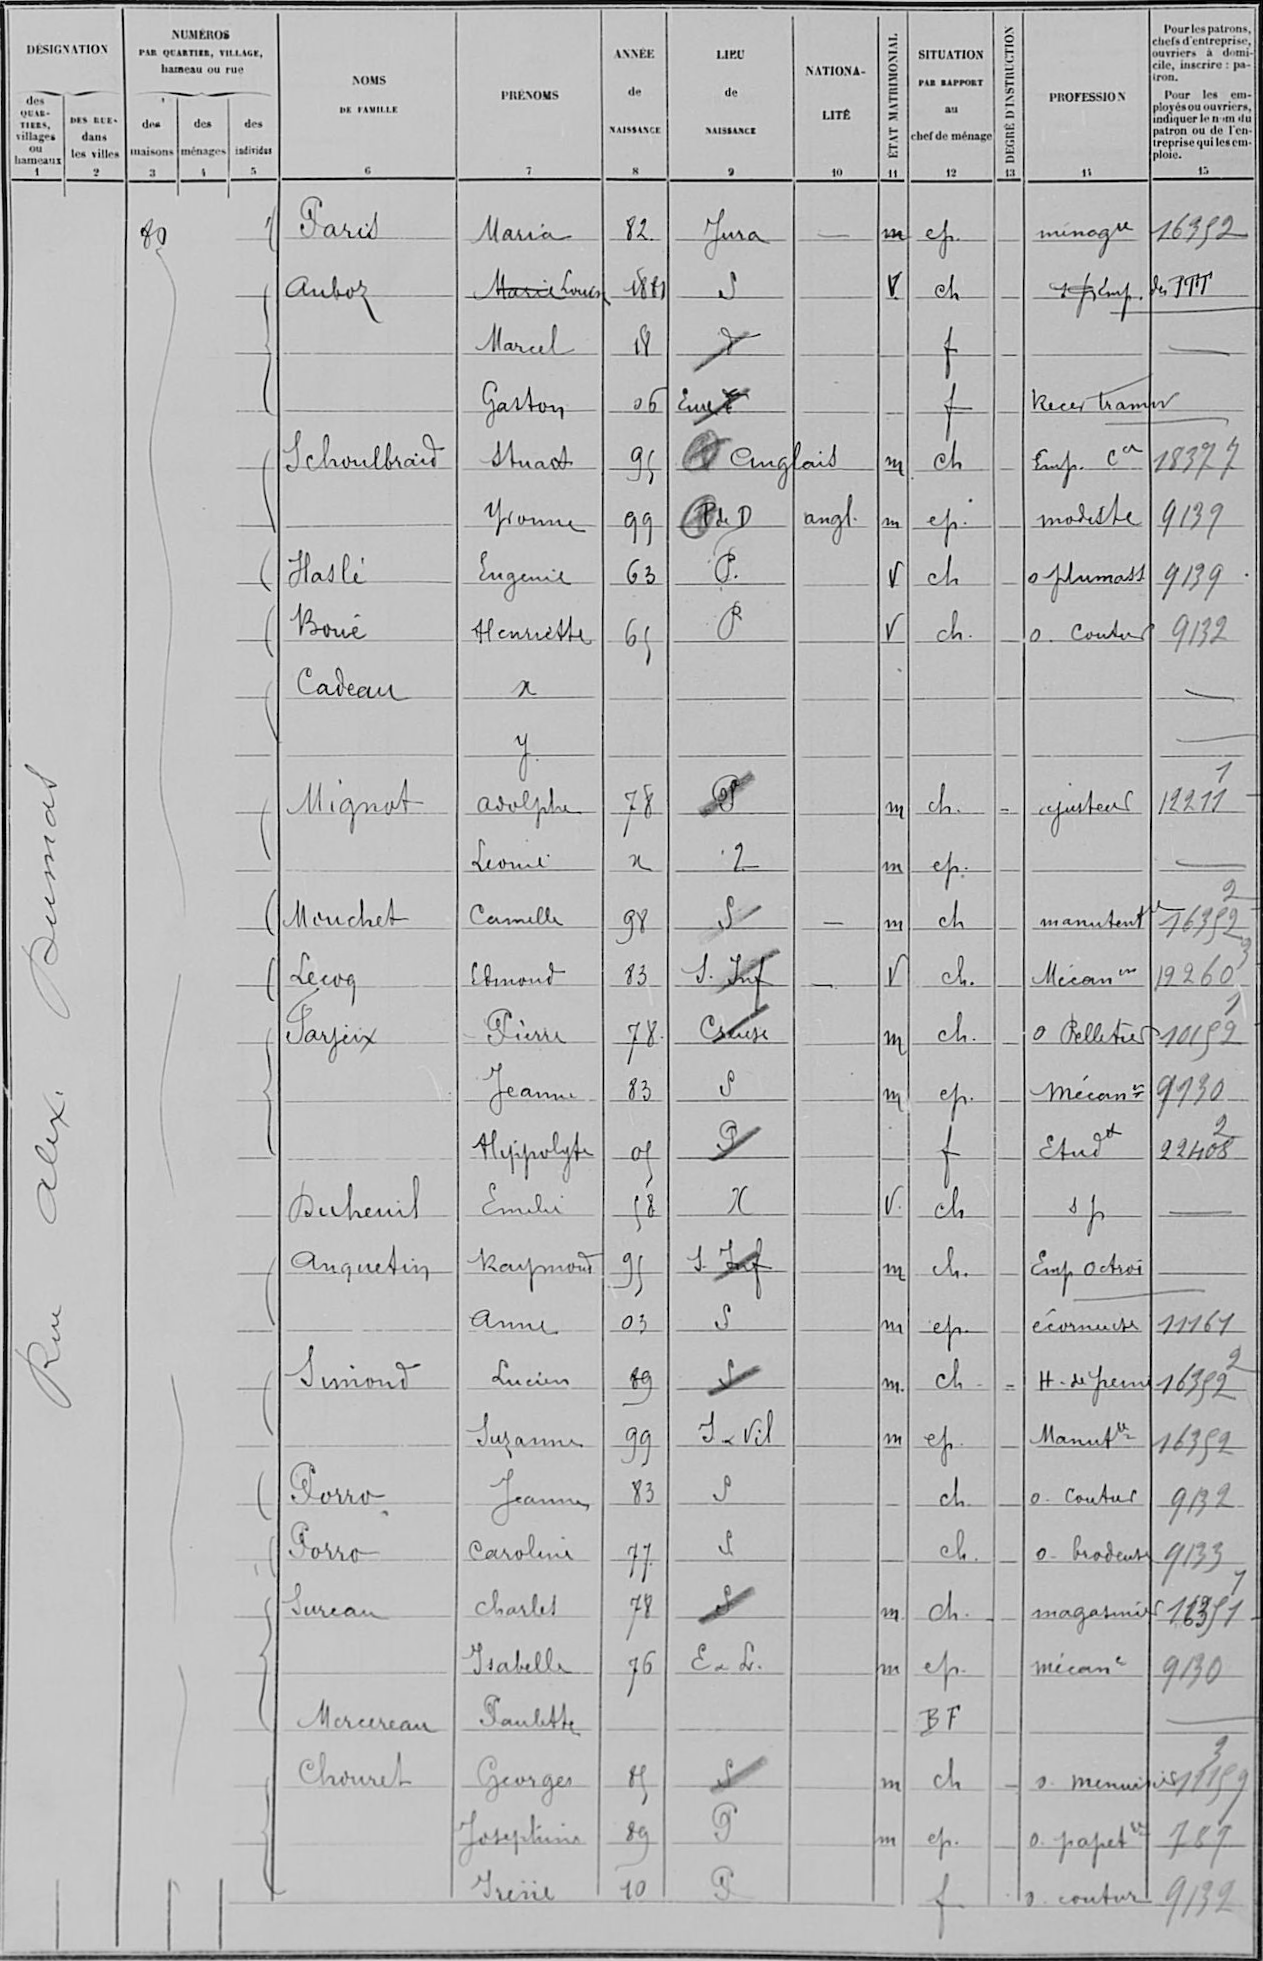Paris/Maria/82/Jura/¤/m/ep/¤/ménagre/16352
Aubor/Marie Louise/1881?/S/¤/V/ch/¤/Emp /des PTT
¤/Marcel/18/S/¤/¤/f/¤/¤/¤
¤/Gaston/06/Eure/¤/¤/f/¤/Recev Tramw/¤
Schoulbraid/Stuart/95/Anglais/¤/m/ch/¤/Emp Cal/18377
¤/Yvonne/99/P de D/angl /m/ep/¤/modiste/9139
Haslé/Eugenie/63/S /¤/V/ch/¤/o plumass/9139
Boué/Henriette/65/S/¤/V/ch /¤/O coutur/9132
Cadeau/x/¤/¤/¤/¤/¤/¤/¤/¤
¤/y/¤/¤/¤/¤/¤/¤/¤/¤
Mignot/Adolphe/78/S/¤/m/ch/¤/ajusteur/12211
¤/Leonie/x/S/¤/m/ep/¤/¤/¤
Mouchet/Camille/98/S/¤/m/ch/¤/manutent/16352
Lecoq/Edmond/83/S Inf/¤/V/ch /¤/Mécan/19260
Sarjeix/Pierre/78/Creuse/¤/m/ch/¤/O Pelletier/10152
¤/Jeanne/83/S/¤/m/ep /¤/mécan/9130
¤/Hippolyte/05/S/¤/¤/f/¤/Etudt/22408
Duheuil/Emilie/58/X/¤/V/Ch/¤/sp/¤
Anquetin/Raymond/95/S Inf/¤/m/ch /¤/Emp Octroi/¤
¤/Anne/03/S/¤/m/ep /¤/écorneuse/11161
Simond/Lucien/89/S/¤/m/ch /¤/H de guerre/16352
¤/Suzanne/99/I&Vil/¤/m/ep /¤/Manut/16352
Gorro/Jeanne/83/S/¤/¤/ch /¤/O Coutur/9132
Gorro/Caroline/77/S/¤/¤/ch/¤/O brodeuse/9133
Sureau/Charles/78/S/¤/m/ch /¤/magasinier/16371
¤/Isabelle/76/E&L/¤/m/ep/¤/mécan/9130
Mercereau/Paulette/¤/¤/¤/¤/BF/¤/¤/¤
Chouret/Georges/85/S/¤/m/ch/¤/o menuisier/18189
¤/Joséphine/89/S/¤/m/ep /¤/o papet /789
¤/Irène/10/S/¤/f/¤/¤/o coutur/9132
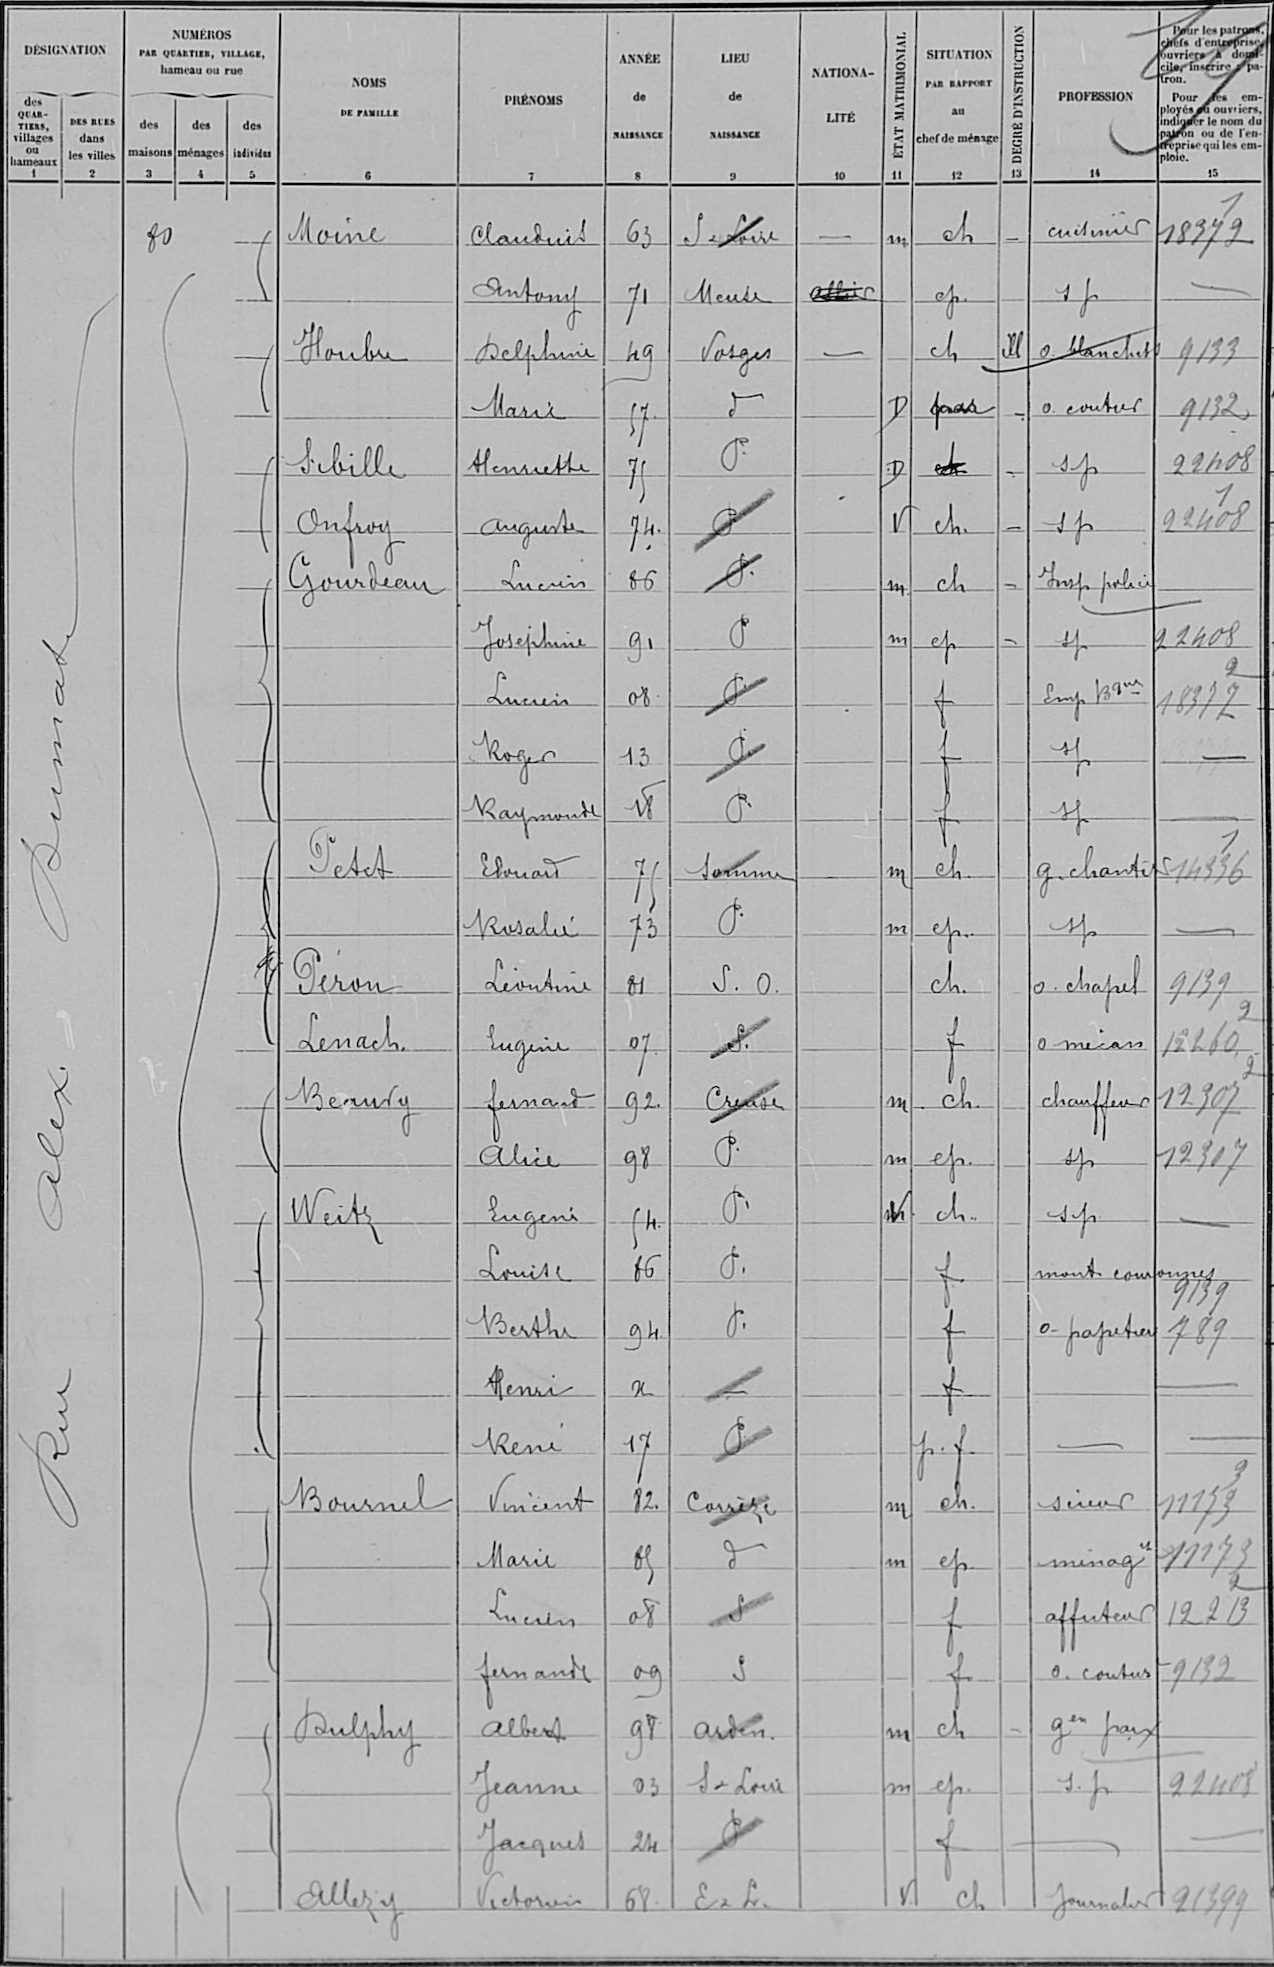Moine/Claudius/63/S&Loire/¤/m/Ch/¤/Cuisinier/18372
¤/Antony/71/Meuse/¤/¤/ep/¤/sp/¤
Houbu/Delphine/49/Vosges/¤/¤/Ch/ill/o blanchiss/9133
¤/Marie/57/d°/¤/D/par/¤/o coutur/9132
Sibille/Henriette/75/P /¤/D/ch /¤/sp/22408
Onfray/auguste/74/P /¤/V/ch /¤/sp/22408
Gourdeau/Lucien/86/P /¤/m/ch/¤/insp police/¤
¤/Joséphine/91/P/¤/m/ep/¤/sp/22408
¤/Lucien/08/P /¤/¤/f/¤/emp Bque/18372
¤/Roger/13/P /¤/¤/f/¤/sp/¤
¤/Raymonde/18/P /¤/¤/f/¤/sp/¤
Peses/Edouard/75/somme/¤/m/ch /¤/g chantier/14356
¤/Rosalie/73/P /¤/m/ep/¤/sp/¤
Péron/Léontine/01/S O /¤/¤/ch /¤/o chapel/9139
Lenach/Eugène/07/P /¤/¤/f/¤/o mecan/12260
Beauvy/Fernand/92/Creuse/¤/m/ch /¤/chauffeur/12307
¤/Alice/98/P /¤/m/ep /¤/sp/12307
Weitz/Eugeni/54/P /¤/V/ch /¤/sp/¤
¤/Louise/86/P /¤/¤/f/¤/mont couronnes/!9139
¤/Berthe/94/P /¤/¤/f/¤/o papetier/789
¤/Henri/x/¤/¤/¤/f/¤/¤/¤
¤/Remi/17/P /¤/¤/fr f /¤/¤/¤
Bournel/Vincent/82/Corrèze/¤/m/ch /¤/sireur/11173
Bournel/marie/85/S/¤/m/ep/¤/ménag/11173
¤/Lucien/08/S/¤/¤/f/¤/affuteur/12213
¤/fernande/09/S/¤/¤/f/¤/o coutur/9132
Dulphy/Albert/98/arden /¤/m/ch/¤/gen fraix/¤
¤/Jeanne/03/S&Loire/¤/m/ep /¤/s p/22408
¤/Jacques/24/S/¤/¤/f/¤/¤/¤
Allery/Victorine/68/¤/¤/V/Ch /¤/Journalier/21399